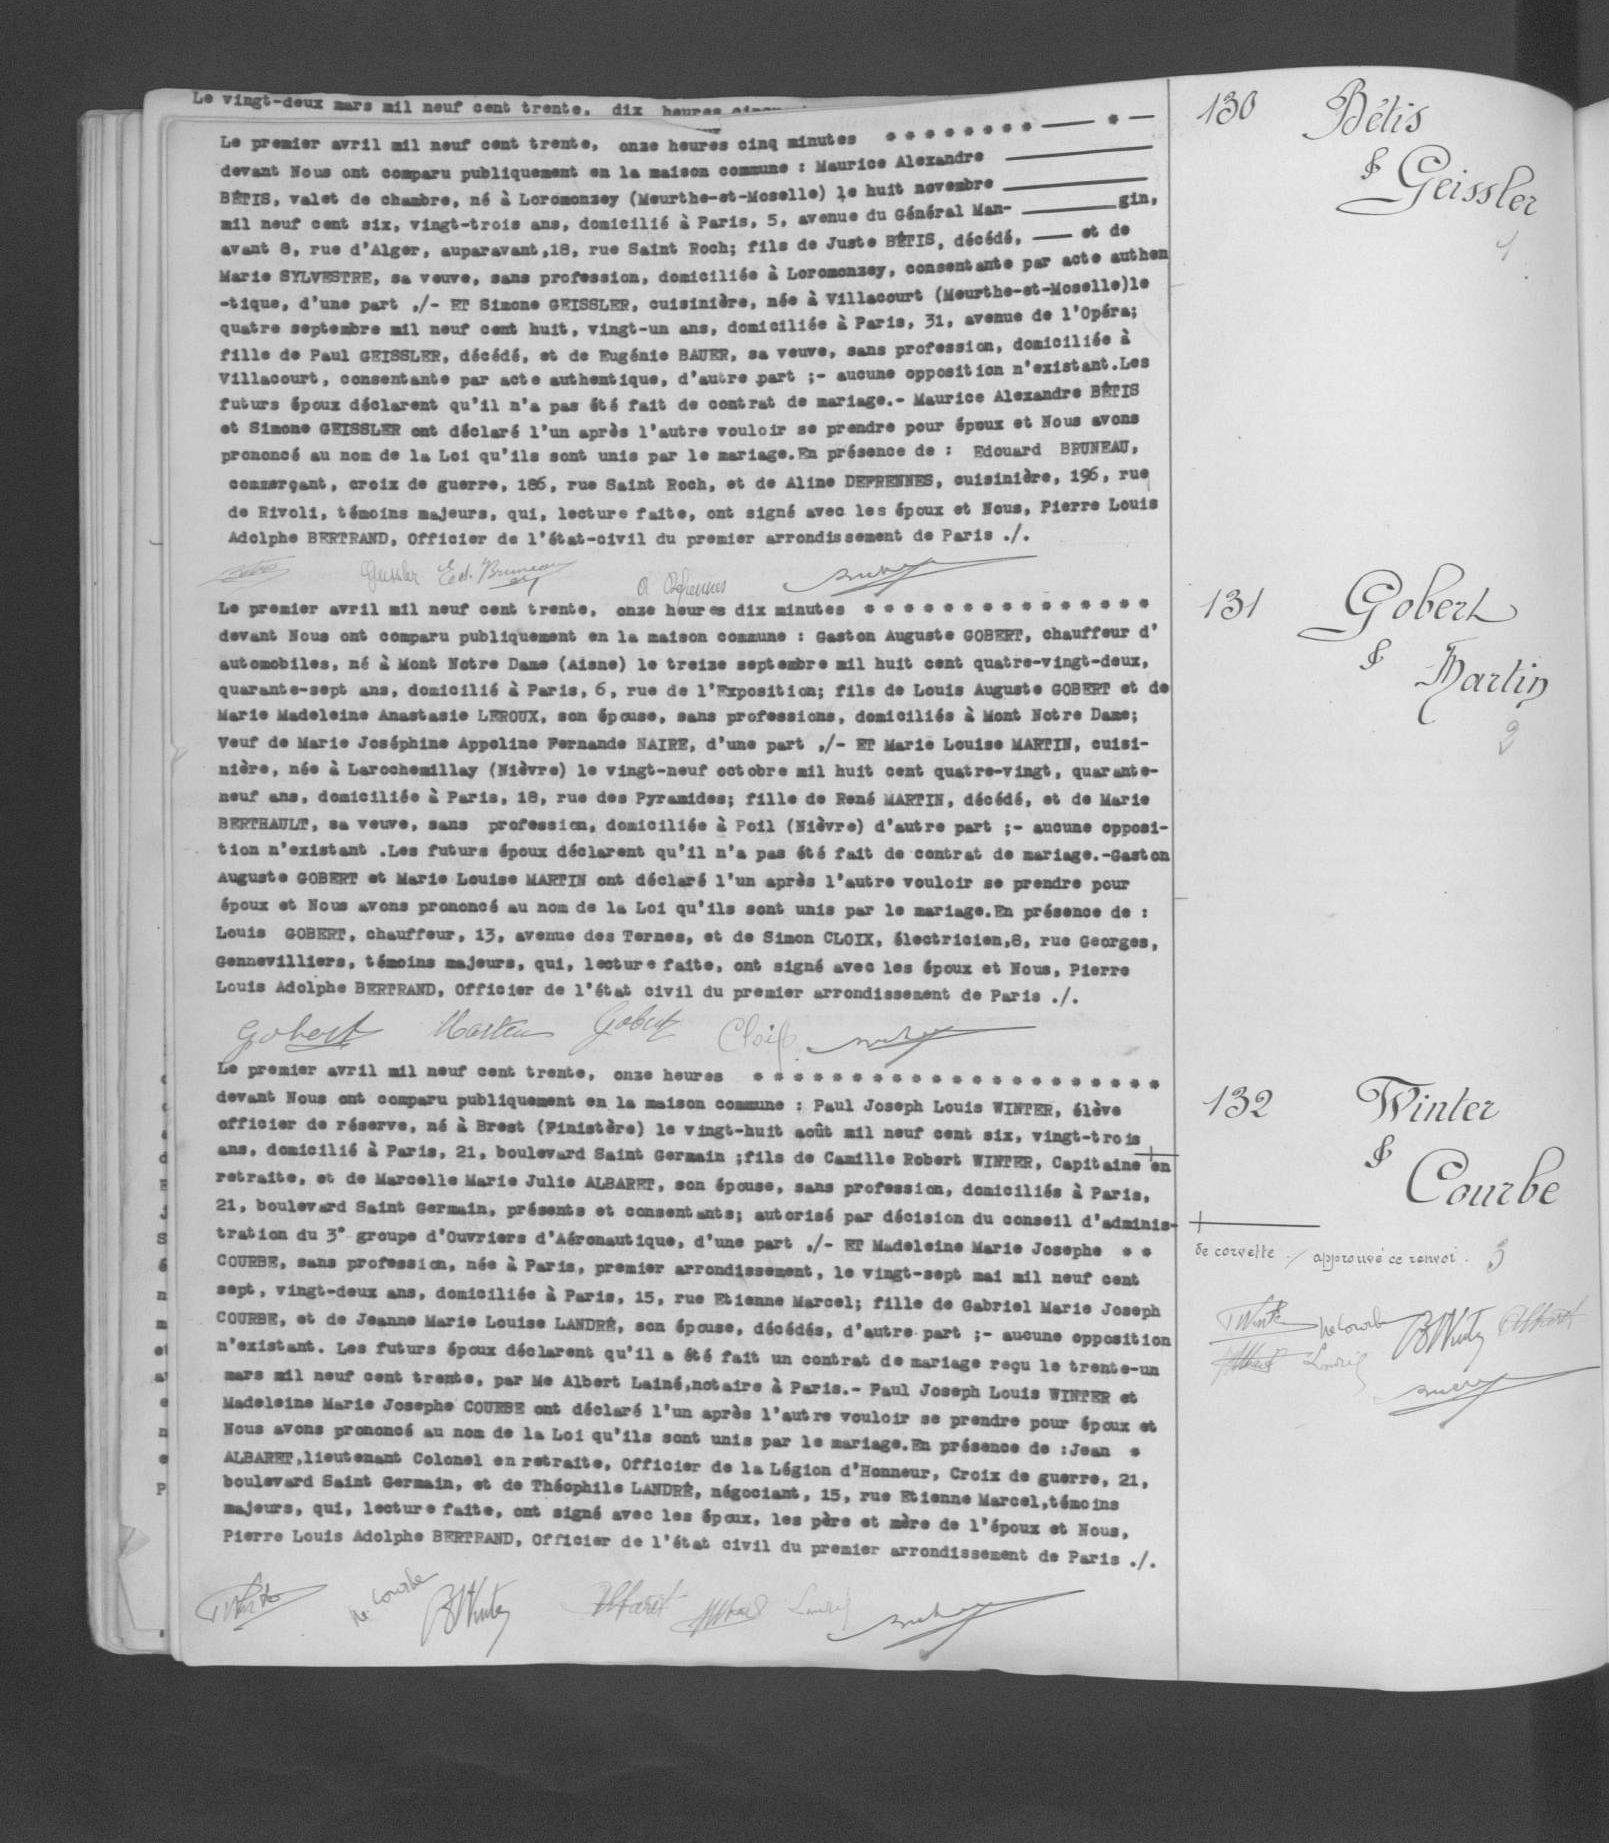Le premier avril mil neuf cent trente, onze heures cinq minutes ****-*-
devant Nous ont comparu publiquement en la maison commune: Maurice Alexandre -
BETIS, valet de chambre, né à Loromonzey (Meurthe-et-Moselle) le huit novembre -
mil neuf cent six, vingt-trois ans, domicilié à Paris, 5, avenue du Général Man-gin,
avant 8, rue d'Alger, auparavant, 18, rue Saint Roch; fils de Juste BETIS, décédé, -et de
Marie SYLVESTRE, sa veuve, sans profession, domiciliée à Loromonzey, consentante par acte authen
-tique, d'une part ,/-ET Simone GEISSLER, cuisinière, née à Villecourt (Meurthe-et-Moselle)le
quatre septembre mil neuf cent huit, vingt-un ans, domiciliée à Paris, 31, avenue de l'Opéra;
fille de Paul GEISSLER, décédé, et de Eugénie BAUER, sa veuve, sans profession, domiciliée à
Villacourt, consentante par acte authentique, d'autre part ;-aucune opposition n'existant.Les
futurs époux déclarent qu'il n'a pas été fait de contrat de mariage .-Maurice Alexandre BETIS
et Simone GEISSLER ont déclaré l'un après l'autre vouloir se prendre pour époux et Nous avons
prononcé au nom de la Loi qu'ils sont unis par le mariage. En présence de: Edouard BRUNEAU,
commerçant, croix de guerre, 186, rue Saint Roch, et de Aline DEFRENNES, cuisinière, 196, rue
de Rivoli, témoins majeurs, qui, lecture faite, ont signé avec les époux et Nous, Pierre Louis
Adolphe BERTRAND, Officier de l'état-civil du premier arrondissement de Paris ./.
Le premier avril mil neuf cent trente, onze heures dix minutes ****
devant Nous ont comparu publiquement en la maison commune: Gaston Auguste GOBERT, chauffeur d'
automobiles, né à Mont Notre Dame (Aisne) le treize septembre mil huit cent quatre-vingt-deux,
quarante-sept ans, domicilié à Paris, 6, rue de l'Exposition; fils de Louis Auguste GOBERT et de
Marie Madeleine Anastasie LEROUX, son épouse, sans profession, domiciliés à Mont Notre Dame;
Veuf de Marie Joséphine Appoline Fernande NAIRE, d'une part ,/-ET Marie Louise MARTIN, cuisi-
nière, née à Larochemillay (Nièvre) le vingt-neuf octobre mil huit cent quatre-vingt, quarante-
neuf ans, domiciliée à Paris, 18, rue des Pyramides; fille de René MARTIN, décédé, et de Marie
BERTHAULT, sa veuve, sans profession, domiciliée à Poil (Nièvre) d'autre part ;-aucune opposi-
tion n'existant. Les futurs époux déclarent qu'il n'a pas été fait de contrat de mariage .-Gaston
Auguste GOBERT et Marie Louise MARTIN ont déclaré l'un après l'autre vouloir se prendre pour
époux et Nous avons prononcé au nom de la Loi qu'ils sont unis par le mariage. En présence de:
Louis GOBERT, chauffeur, 13, avenue des Ternes, et de Simon CLOIX, électricien, 8, rue Georges,
Gennevilliers, témoins majeurs, qui, lecture faite, ont signé avec les époux et Nous, Pierre
Louis Adolphe BERTRAND, Officier de l'état civil du premier arrondissement de Paris ./.
Le premier avril mil neuf cent trente, onze heures ****
devant Nous ont comparu publiquement en la maison commune: Paul Joseph Louis WINTER, élève
officier de réserve, né à Brest (Finistère) le vingt-huit août mil neuf cent six, vingt-trois
ans, domicilié à Paris, 21, boulevard Saint Germain; fils de Camille Robert WINTER, Capitaine en
retraite, et de Marcelle Marie Julie ALBARET, son épouse, sans profession domiciliés à Paris,
21, boulevard Saint Germain, présents et consentants; autorisé par décision du conseil d'adminis-
tration du 3° groupe d'Ouvriers d'Aéronautique, d'une part ,/-ET Madeleine Marie Josephe * *
COURBE, sans profession, née à Paris, premier arrondissement, le vingt-sept mai mil neuf cent
sept, vingt-deux ans, domiciliée à Paris, 15, rue Etienne Marcel; fille de Gabriel Marie Joseph
COURBE, et de Jeanne Marie Louise LANDRE, son épouse, décédés, d'autre part ;-aucune opposition
n'existant. Les futurs époux déclarent qu'il a été fait un contrat de mariage reçu le trente-un
mars mil neuf cent trente, par Me Albert Lainé, notaire à Paris .-Paul Joseph Louis WINTER et
Madeleine Marie Josephe COURBE ont déclaré l'un après l'autre vouloir se prendre pour époux et
Nous avons prononcé au nom de la Loi qu'ils sont unis par le mariage. En présence de: Jean *
ALBARET, lieutenant Colonel en retraite, Officier de la Légion d'Honneur, Croix de guerre, 21,
boulevard Saint Germain, et de Théophile LANDRE, négociant, 15, rue Etienne Marcel, témoins
majeurs, qui, lecture faite, ont signé avec les époux, les père et mère de l'époux et Nous,
Pierre Louis Adolphe BERTRAND, Officier de l'état civi du premier arrondissement de Paris ./.
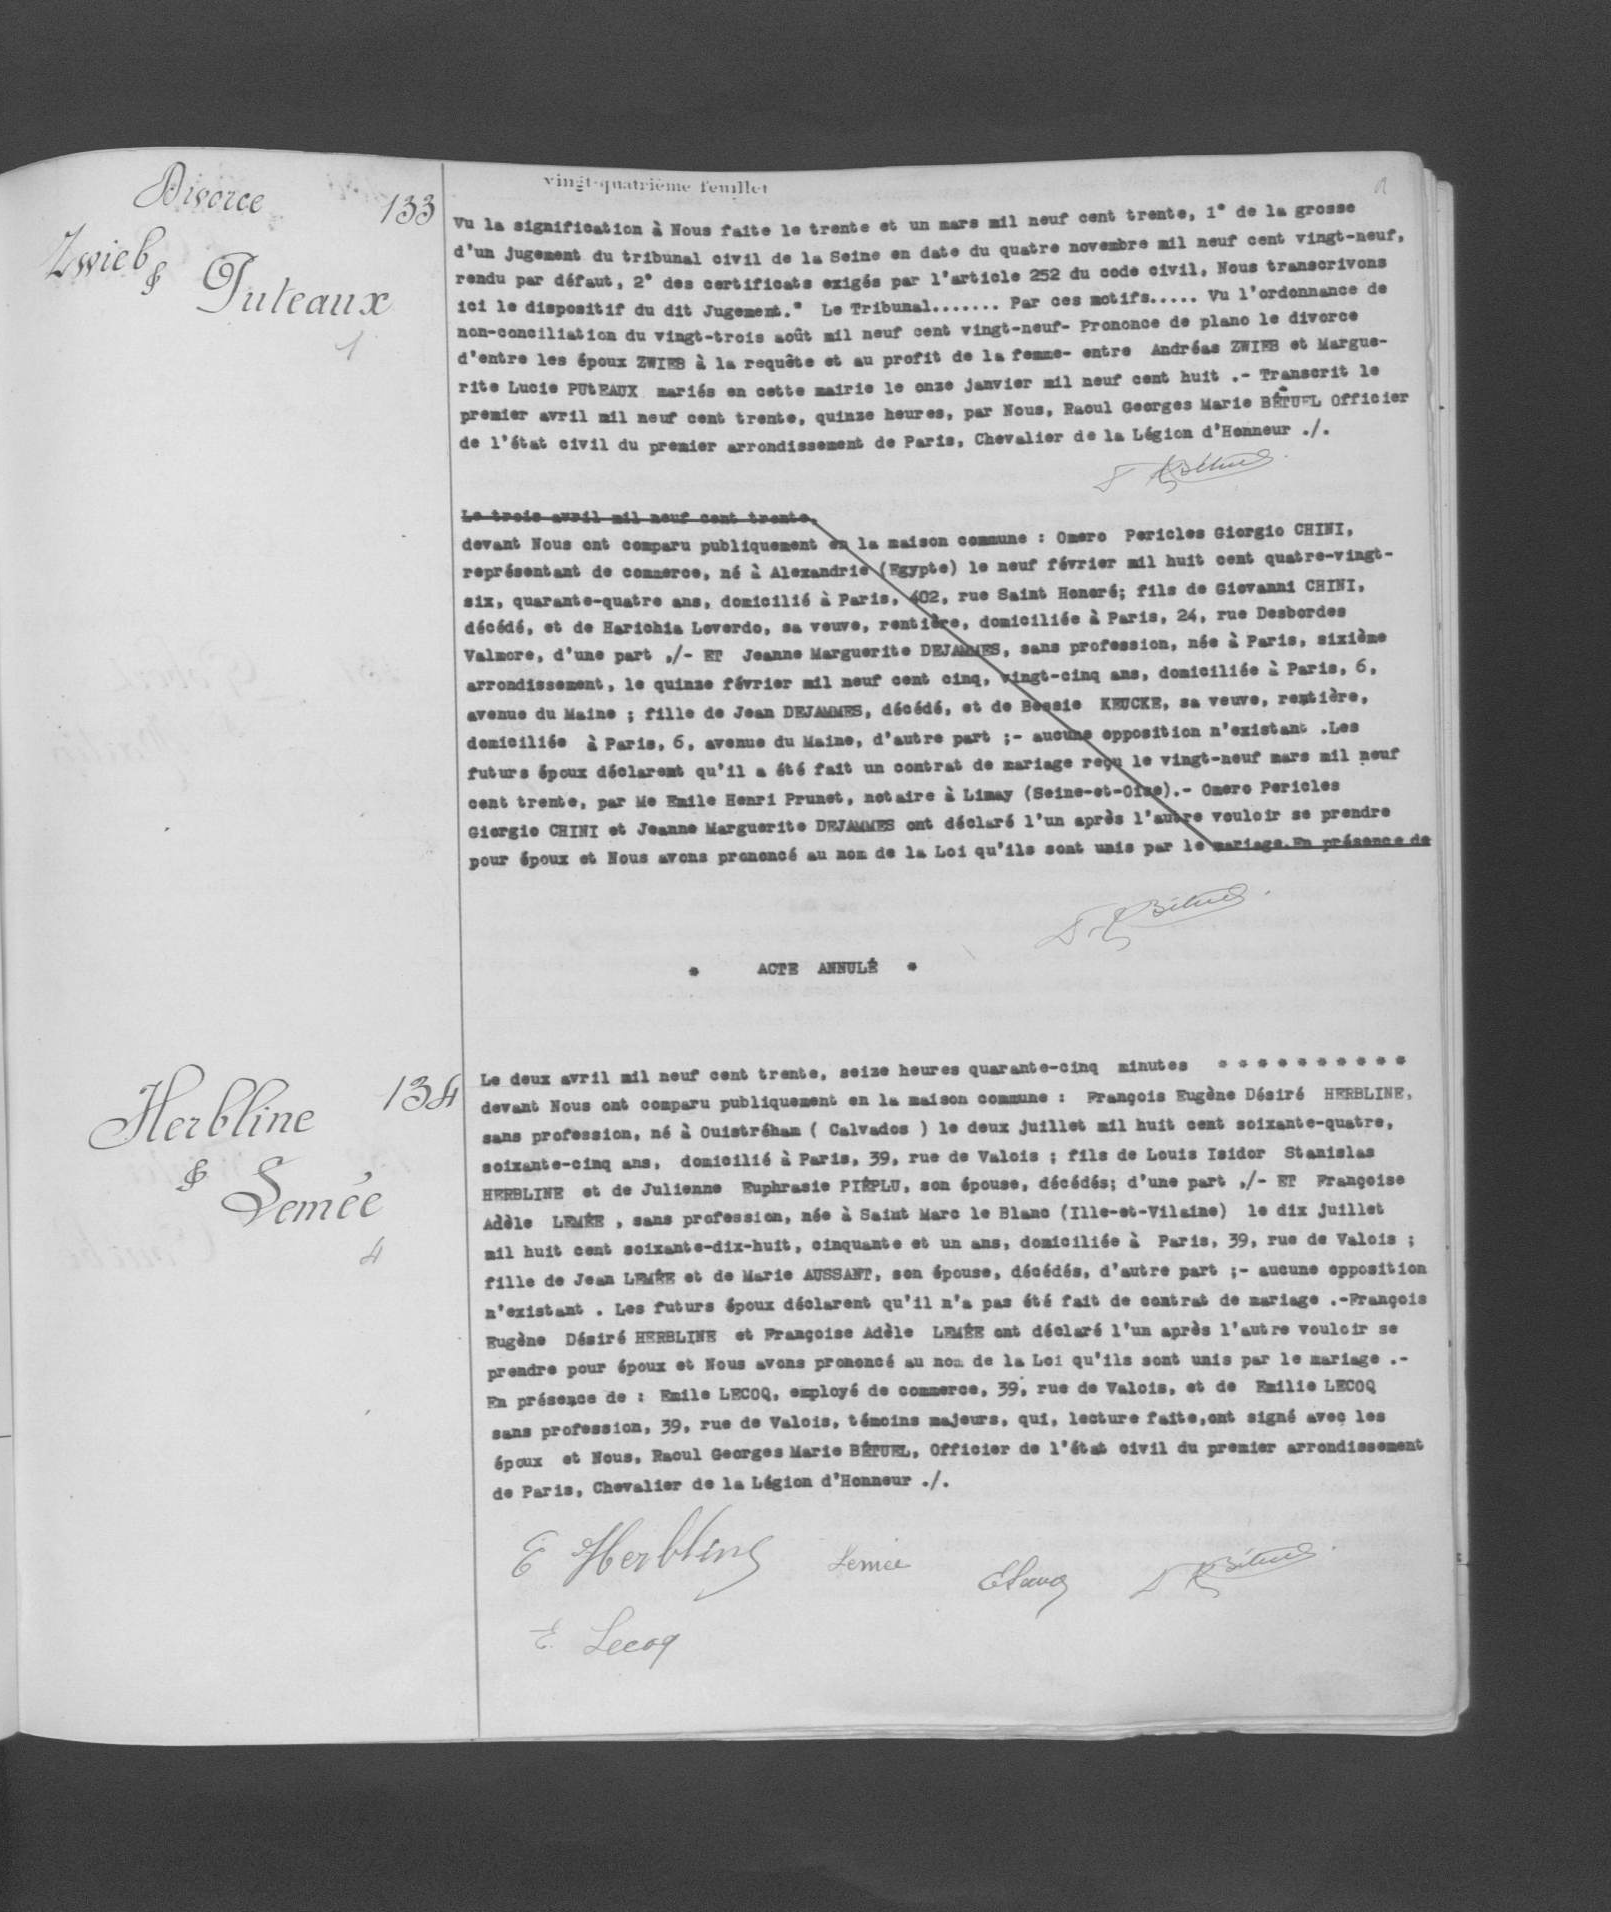Vu la signification à Nous faite le trente et un mars mil neuf cent trente, 1° de la grosse
d'un jugement du tribunal civil de la Seine en date du quatre novembre mil neuf cent vingt-neuf,
rendu par défaut, 2° des certificats exigés par l'article 252 du code civil, Nous transcrivons
ici le dispositif du dit Jugement." Le Tribunal..... Par ces motifs..... Vu l'ordonnance de
non-conciliation du vingt-trois août mil neuf cent vingt-neuf-Prononce de plano le divorce
d'entre les époux ZWIEB à la requête et au profit de la femme-entre Andréas ZWIEB et Margue-
rite Lucie PUtEAUX mariés en cette mairie le onze janvier mil neuf cent huit .-Transcrit le
premier avril mil neuf cent trente, quinze heures, par Nous, Raoul Georges Marie BETUEL Officier
de l'état civil du premier arrondissement de Paris, Chevalier de la Légion d'Honneur ./.
Le trois avril mil neuf cent trente,
devant Nous ont comparu publiquement en la maison commune: Omero Pericles Giorgio CHINI,
représentant de commerce, né à Alexandrie (Egypte) le neuf février mil huit cent quatre-vingt-
six, quarante-quatre ans, domicilié à Paris, 402, rue Saint Honoré; fils de Giovanni CHINI,
décédé, et de Harichia Loverdo, sa veuve, rentière, domiciliée à Paris, 24, rue Desbordes
Valmore, d'une part ,/-ET Jeanne Marguerite DEJAMMES, sans profession, née à Paris, sixième
arrondissement, le quinze février mil neuf cent cinq, vingt-cinq ans, domiciliée à Paris, 6,
avenue du Maine; fille de Jean DEJAMMES, décédé, et de Bessie KEUCKE, sa veuve, rentière,
domiciliée à Paris, 6, avenue du Maine, d'autre part ;-aucune opposition n'existant .Les
futurs époux déclarent qu'il a été fait un contrat de mariage reçu le vingt-neuf mars mil neuf
cent trente, par Me Emile Henri, Prunet, notaire à Limay (Seine-et-Oise) .-Omeo Pericles
Giorgio CHINI et Jeanne Marguerite DEJAMMES ont déclaré l'un après l'autre vouloir se prendre
pour époux et Nous avons prononcé au nom de la Loi qu'ils sont unis par le mariage. En présence de
* ACTE ANNULE *
Le deux avril mil neuf cent trente, seize heures quarante-cinq minutes ****
devant Nous ont comparu publiquement en la maison commune: François Eugène Désiré HERBLINE,
sans profession, né à Ouistréham (Calvados) le deux juillet mil huit cent soixante-quatre,
soixante-cinq ans, domicilié à Paris, 39, rue de Valois; fils de Louis Isidor Stanislas
HERBLINE et de Julienne Euphrasie PIEPLU, son épouse, décéés; d'une part ,/-ET Françoise
Adèle LEMEE, sans profession, née à Saint Marc le Blanc (Ille-et-Vilaine) le dix juillet
mil huit cent soixante-dix-huit, cinquante et un ans, domiciliée à Paris, 39, rue de Valois;
fille de Jean LEMEE et de Marie AUSSANT, son épouse, décédés, d'autre part ;-aucune opposition
n'existant. Les futurs époux déclarent qu'il n'a pas été fait de contrat de mariage .-François
Eugène Désiré HERBLINE et Françoise Adèle LEMEE ont déclaré l'un après l'autre vouloir se
prendre pour époux et Nous avons prononcé au nom de la Loi qu'ils sont unis par le mariage .-
En présence de: Emile LECOQ, employé de commerce, 39, rue de Valois, et de Emilie LECOQ
sans profession, 39, rue de Valois, témoins majeurs, qui, lecture faite, ont signé avec les
époux et Nous, Raoul Georges Marie BETUEL, Officier de l'état civil du premier arrondissement
de Paris, Chevalier de la Légion d'Honneur ./.